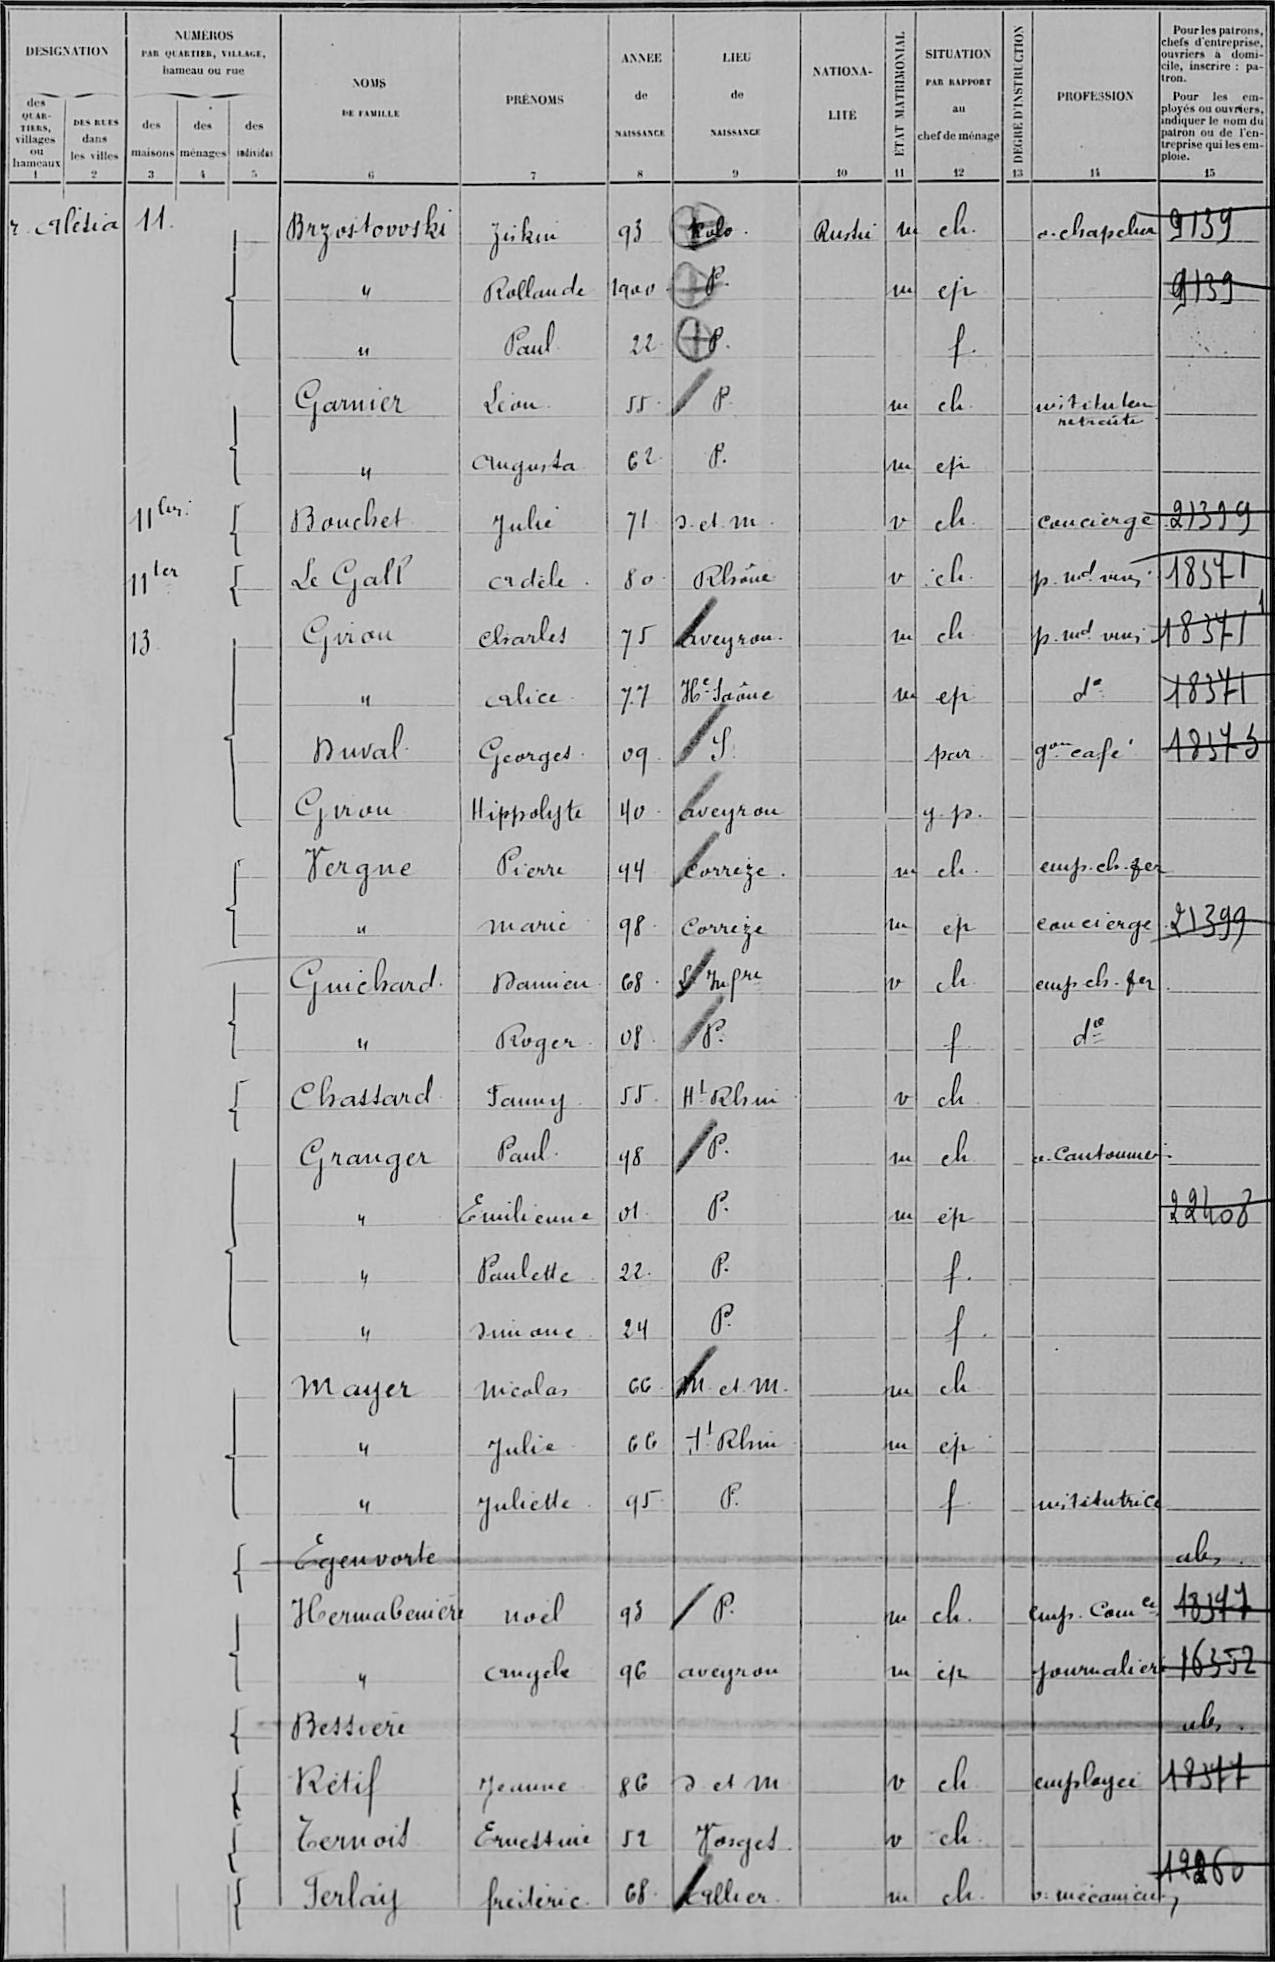Brzostovoski/Ziskin/93/Rulo/Russie/m/ch /¤/o chapelier/9139
"/Rollande/1900/P /¤/m/ep/¤/¤/9139
"/Paul/22/P /¤/¤/f /¤/¤/¤
Garnier/Léon/55/P /¤/m/ch /instituteur retraité/¤/¤
"/Augusta/62/P /¤/m/ep/¤/¤/¤
Bouchet/Julie/71/s et m/¤/v/ch /¤/concierge/21399
Le Gall/Adèle/80/Rhône/¤/v/ch/¤/p md vins/18371
Girou/Charles/75/aveyron/¤/m/ch/¤/p md vins/18371
"/calice/77/Hte-Saône/¤/m/ep/¤/d°/18371
Duval/Georges/09/S/¤/¤/par/¤/gon café/18373
Girou/Hippolyte/40/Aveyron/¤/¤/g p/¤/¤/¤
Vergne/Pierre/94/Corrèze/¤/m/ch /¤/emp ch fer/¤
"/Marie/98/Corrèze/¤/m/ep/¤/concierge/21399
Guichard/Damien/68/L Infre/¤/v/ch/¤/emp ch fer /¤
"/Roger/08/P /¤/¤/f/¤/d°/¤
Chassard/Fanny/55/Ht Rhin/¤/V/ch/¤/¤/¤
Granger/Paul/98/P /¤/m/ch/¤/a Cantonnier/¤
"/Emilienne/01/P /¤/m/ép/¤/¤/22408
"/Paulette/22/P /¤/¤/f /¤/¤/¤
"/Simone/24/P /¤/¤/f /¤/¤/¤
Mayer/Nicolas/66/m et m /¤/m/ch/¤/¤/¤
"/Julie/66/Ht Rhin/¤/m/ép/¤/¤/¤
"/Juliette/95/P /¤/¤/f/¤/institutrice/¤
Egenvorte/¤/¤/¤/¤/¤/¤/¤/¤/abs
Hermabeuière/noel/93/P /¤/m/ch /¤/emp Comce/18397
"/Angele/96/aveyron/¤/m/ép/¤/journaliere/16352
Bessiere/¤/¤/¤/¤/¤/¤/¤/¤/abs
Retif/Jeanne/86/S et m/¤/v/ch/¤/employée/18377
Ternois/Ernestine/52/Vosges/¤/v/ch/¤/¤/12260
Ferlay/frédéric/68/Allier/¤/m/ch /¤/o mécanicien/12260
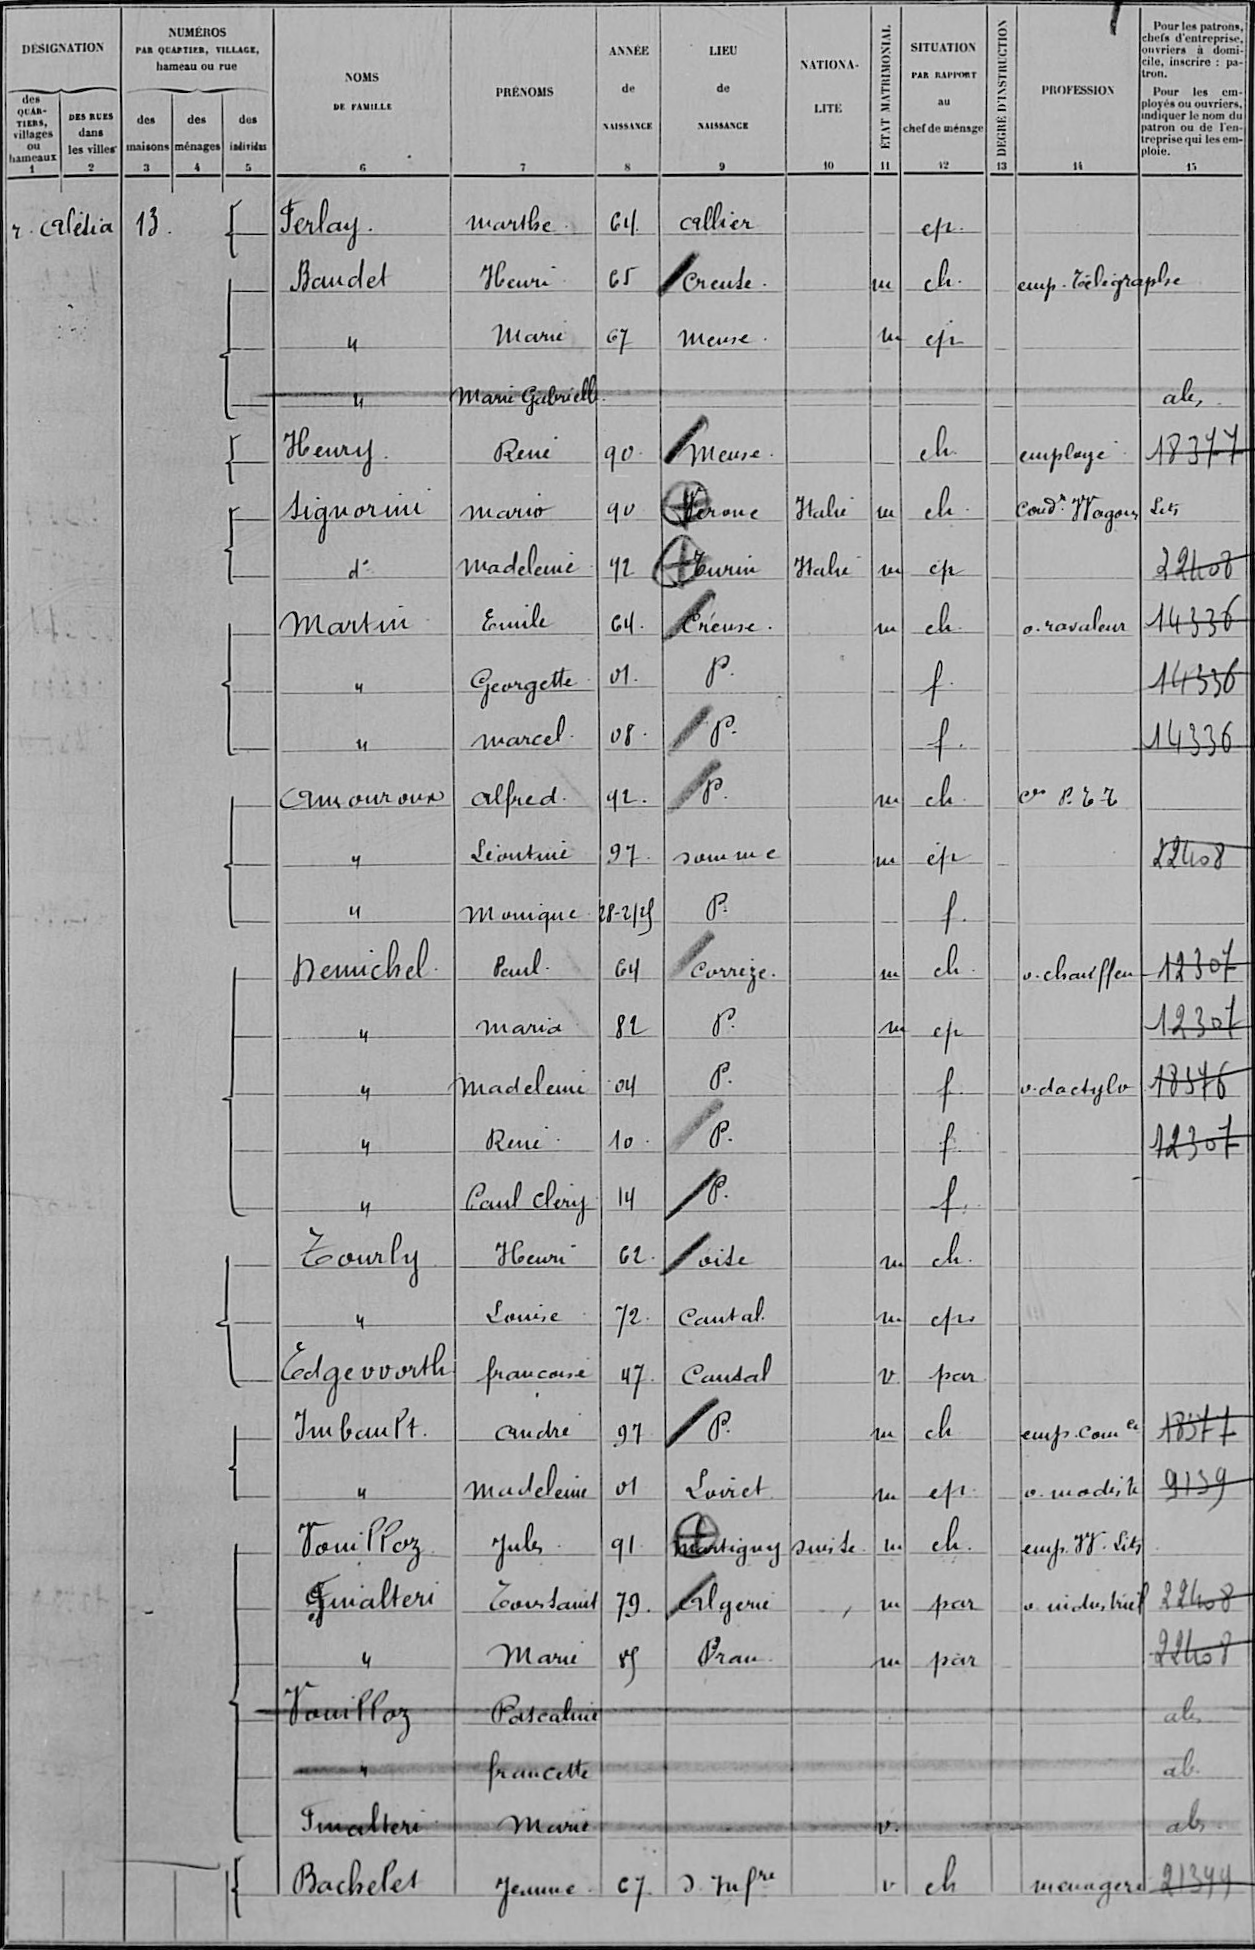Ferlay/marthe/64/Allier/¤/ep /¤/¤/¤/¤
Baudet/Henri/65/Creuse /¤/m/ch /¤/emp télégraphe/¤
''/Marie/67/meuse/¤/m/ép/¤/¤/¤
''/Marie Gabrielle/¤/¤/¤/¤/¤/¤/¤/abs
Henry/René/90/meuse/¤/¤/ch/¤/employé/18377
Signorini/Mario/90/Verone/Italie/m/ch /¤/cond Wagons lits/¤
d°/Madeleine/92/Turin/Italie/m/ep/¤/¤/22408
Martin/Emilie/64/Creuse/¤/m/ch/¤/o ravaleur/14336
''/Georgette/01/P /¤/¤/f /¤/¤/14336
''/Marcel/08/P /¤/¤/f /¤/¤/14336
Amouroux/Alfred/92 /P /¤/m/ch /¤/em PTT/¤
''/Léontine/97/Somme/¤/m/ép/¤/¤/22408
"/Monique/28 2:29/P /¤/¤/f /¤/¤/¤
Demichel/Paul/64/Corrèze /¤/m/ch /¤/o chauffeur/12307
''/Maria/82/P /¤/m/ep/¤/¤/12307
''/Madeleine/04/P /¤/¤/f /¤/o dactylo/18376
''/René/10/P /¤/¤/f/¤/¤/12307
''/Paul Clery/14/P /¤/¤/f /¤/¤/¤
Tourly/Henri/62/oise/¤/m/ch /¤/¤/¤
''/Louise/72/Cantal/¤/m/ep/¤/¤/¤
Edgevvorth/francoise/47/Cantal/¤/v/par/¤/¤/¤
Imbault/André/97/P /¤/m/ch/¤/emp comce/18377
''/madeleine/01/Loiret/¤/m/ep/¤/o modiste/9139
Vouillez/Jules/91/martigny/suisse/m/ch /¤/emp VV Lits/¤
Fuialteri/Toussaint/79/Algerie/¤/m/par/¤/o industriel/22408
''/Marie/89/Drome/¤/m/par/¤/¤/22408
Vouilloz/Pascaline/¤/¤/¤/¤/¤/¤/¤/abs
''/francette/¤/¤/¤/¤/¤/¤/¤/abs
Fuialteri/Marie/¤/¤/¤/v /¤/¤/¤/abs
Bachelet/Jeanne/67/S Infre/¤/V/ch/¤/menagere/21399